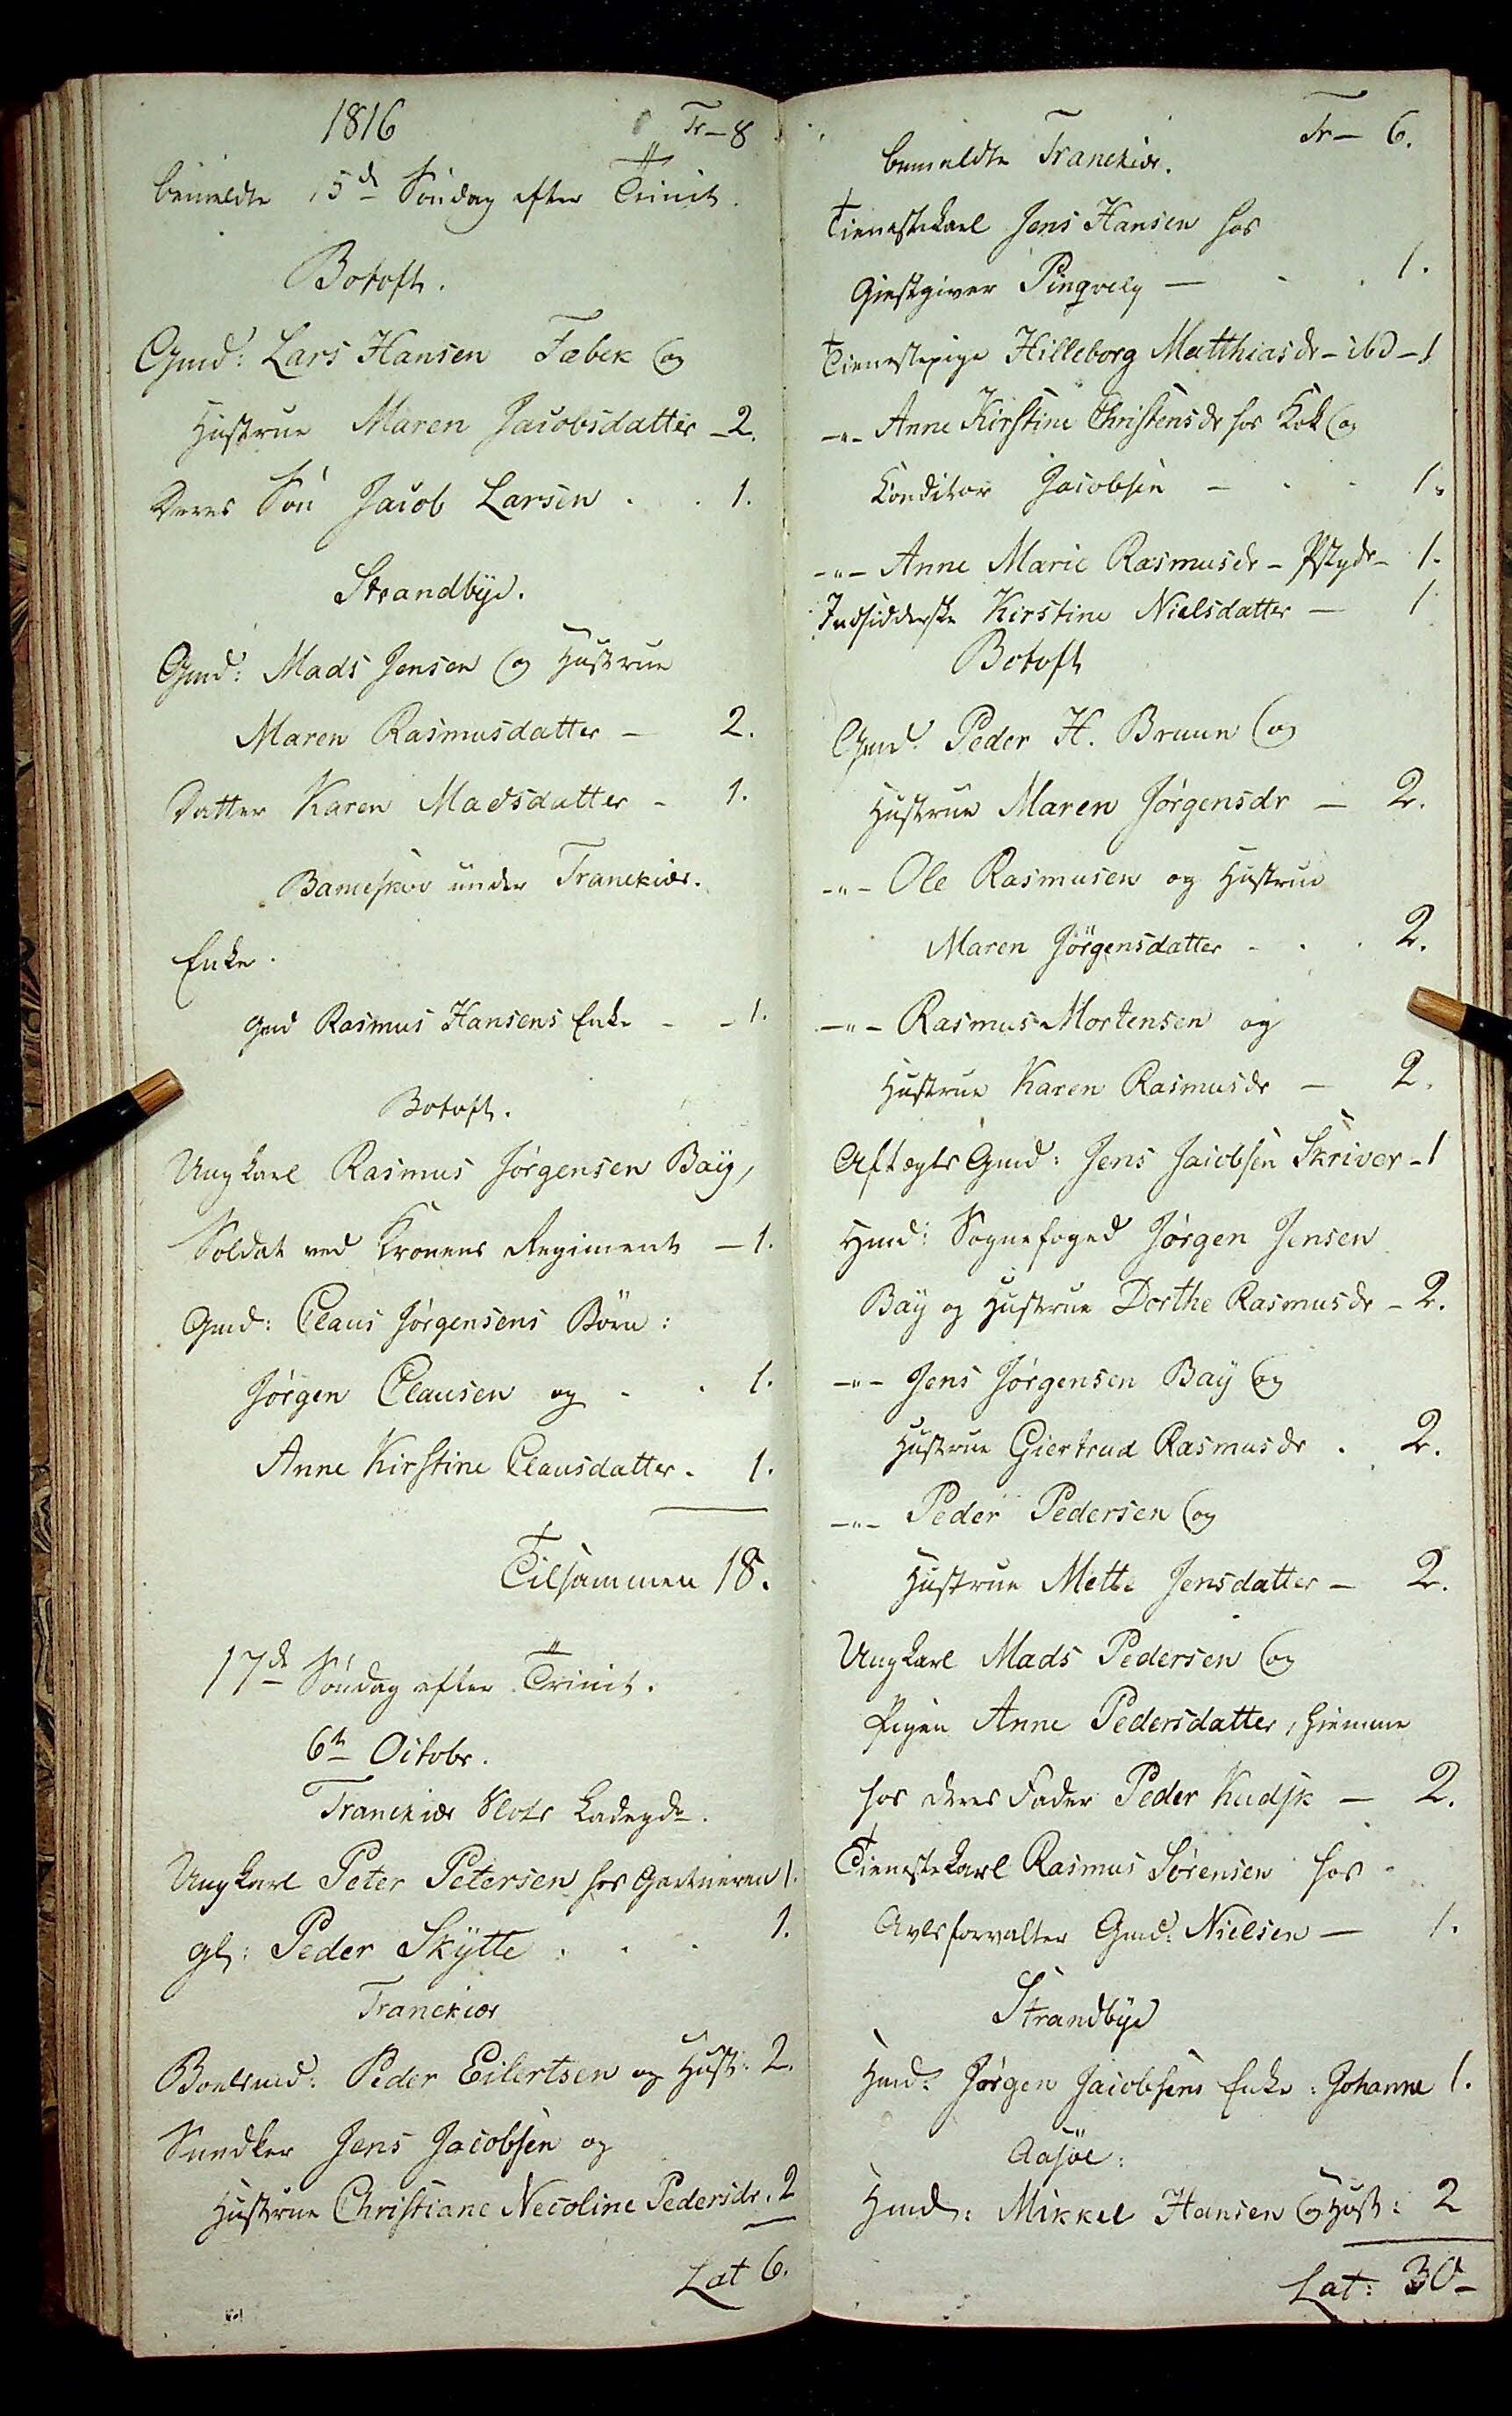1816
Tr - 8
bemeldte 15de Søndag efter Trinit.
Botoft.
Gmd: Lars Hansen Fæbek og
Hustrue Maren Jacobsdatter - 2.
Deres Søn Jacob Larsen . . 1.
Strandbye.
Gmd: Mads Jensen og Hustrue
Maren Rasmusdatter - 2.
Datter Karen Madsdatter - 1.
Bameskov under Tranekiær.
Enke
Gmd Rasmus Hansens Enke - - 1.
Botoft.
Ungkarl Rasmus Jørgensen Bay,
Soldat ved Konens Regiment - 1.
Gmd: Claus Jørgensens Børn:
Jørgen Clausen og . . 1.
Anne Kirstine Clausdatter . 1.
Tilsammen 18.
17de Søndag efter Trinit.
6te Octobr.
Tranekiær Slots Ladegdr
Ungkarl Peter Petersen hos Gartneren 1.
gl: Peder Skytte . . . 1.
Tranekiær
Boelsmd: Peder Eilertsen og Hust . 2.
Snedker Jens Jacobsen og
Hustrue Christiane Nicoline Pedersdr . 2
Lat 6.
Tr - 6.
bemeldte Tranekiær.
Tienestekarl Jens Hansen hos
Giestegiver Pingvely - . . 1.
Tienestepige Hilleborg Matthiasdr - ibd - 1
-"- Anne Kirstine Christensdr hos Kok og
Konditor Jacobsen - . . 1.
-"- Anne Marie Rasmusdr - Pstgdr 1.
Indsidderske Kirstine Nielsdatter - 1
Botoft
Gmd.  Peder H. Bruun og
Hustrue Maren Jørgensdr - 2.
-"- Ole Rasmusen og Hustrue
Maren Jørgensdatter . . . 2.
-"- Rasmus Mortensen og
Hustrue Karen Rasmusdr - 2.
Aftægts Gmd: Jens Jacobsen Skriver - 1
Hmd: Sognefoged Jørgen Jensen
Bay og Hustrue Dorthe Rasmusdr - 2.
-"- Jens Jørgensen Bay og
Hustrue Giertrud Rasmusdr . 2.
-"- Peder Pedersen og
Hustrue Mette Jensdatter - 2.
Ungkarl Mads Pedersen og
Pigen Anne Pedersdatter, hjemme
hos deres Fader Peder Kudsk - 2.
Tienestekarl Rasmus Sørensen hos
Avlsforvalter Gmd: Nielsen - 1.
Strandbye
Hmd: Jørgen Jacobsens Enke: Johanne 1.
Aasøe:
Hmd: Mikkel Hansen og Hust : 2
Lat: 30
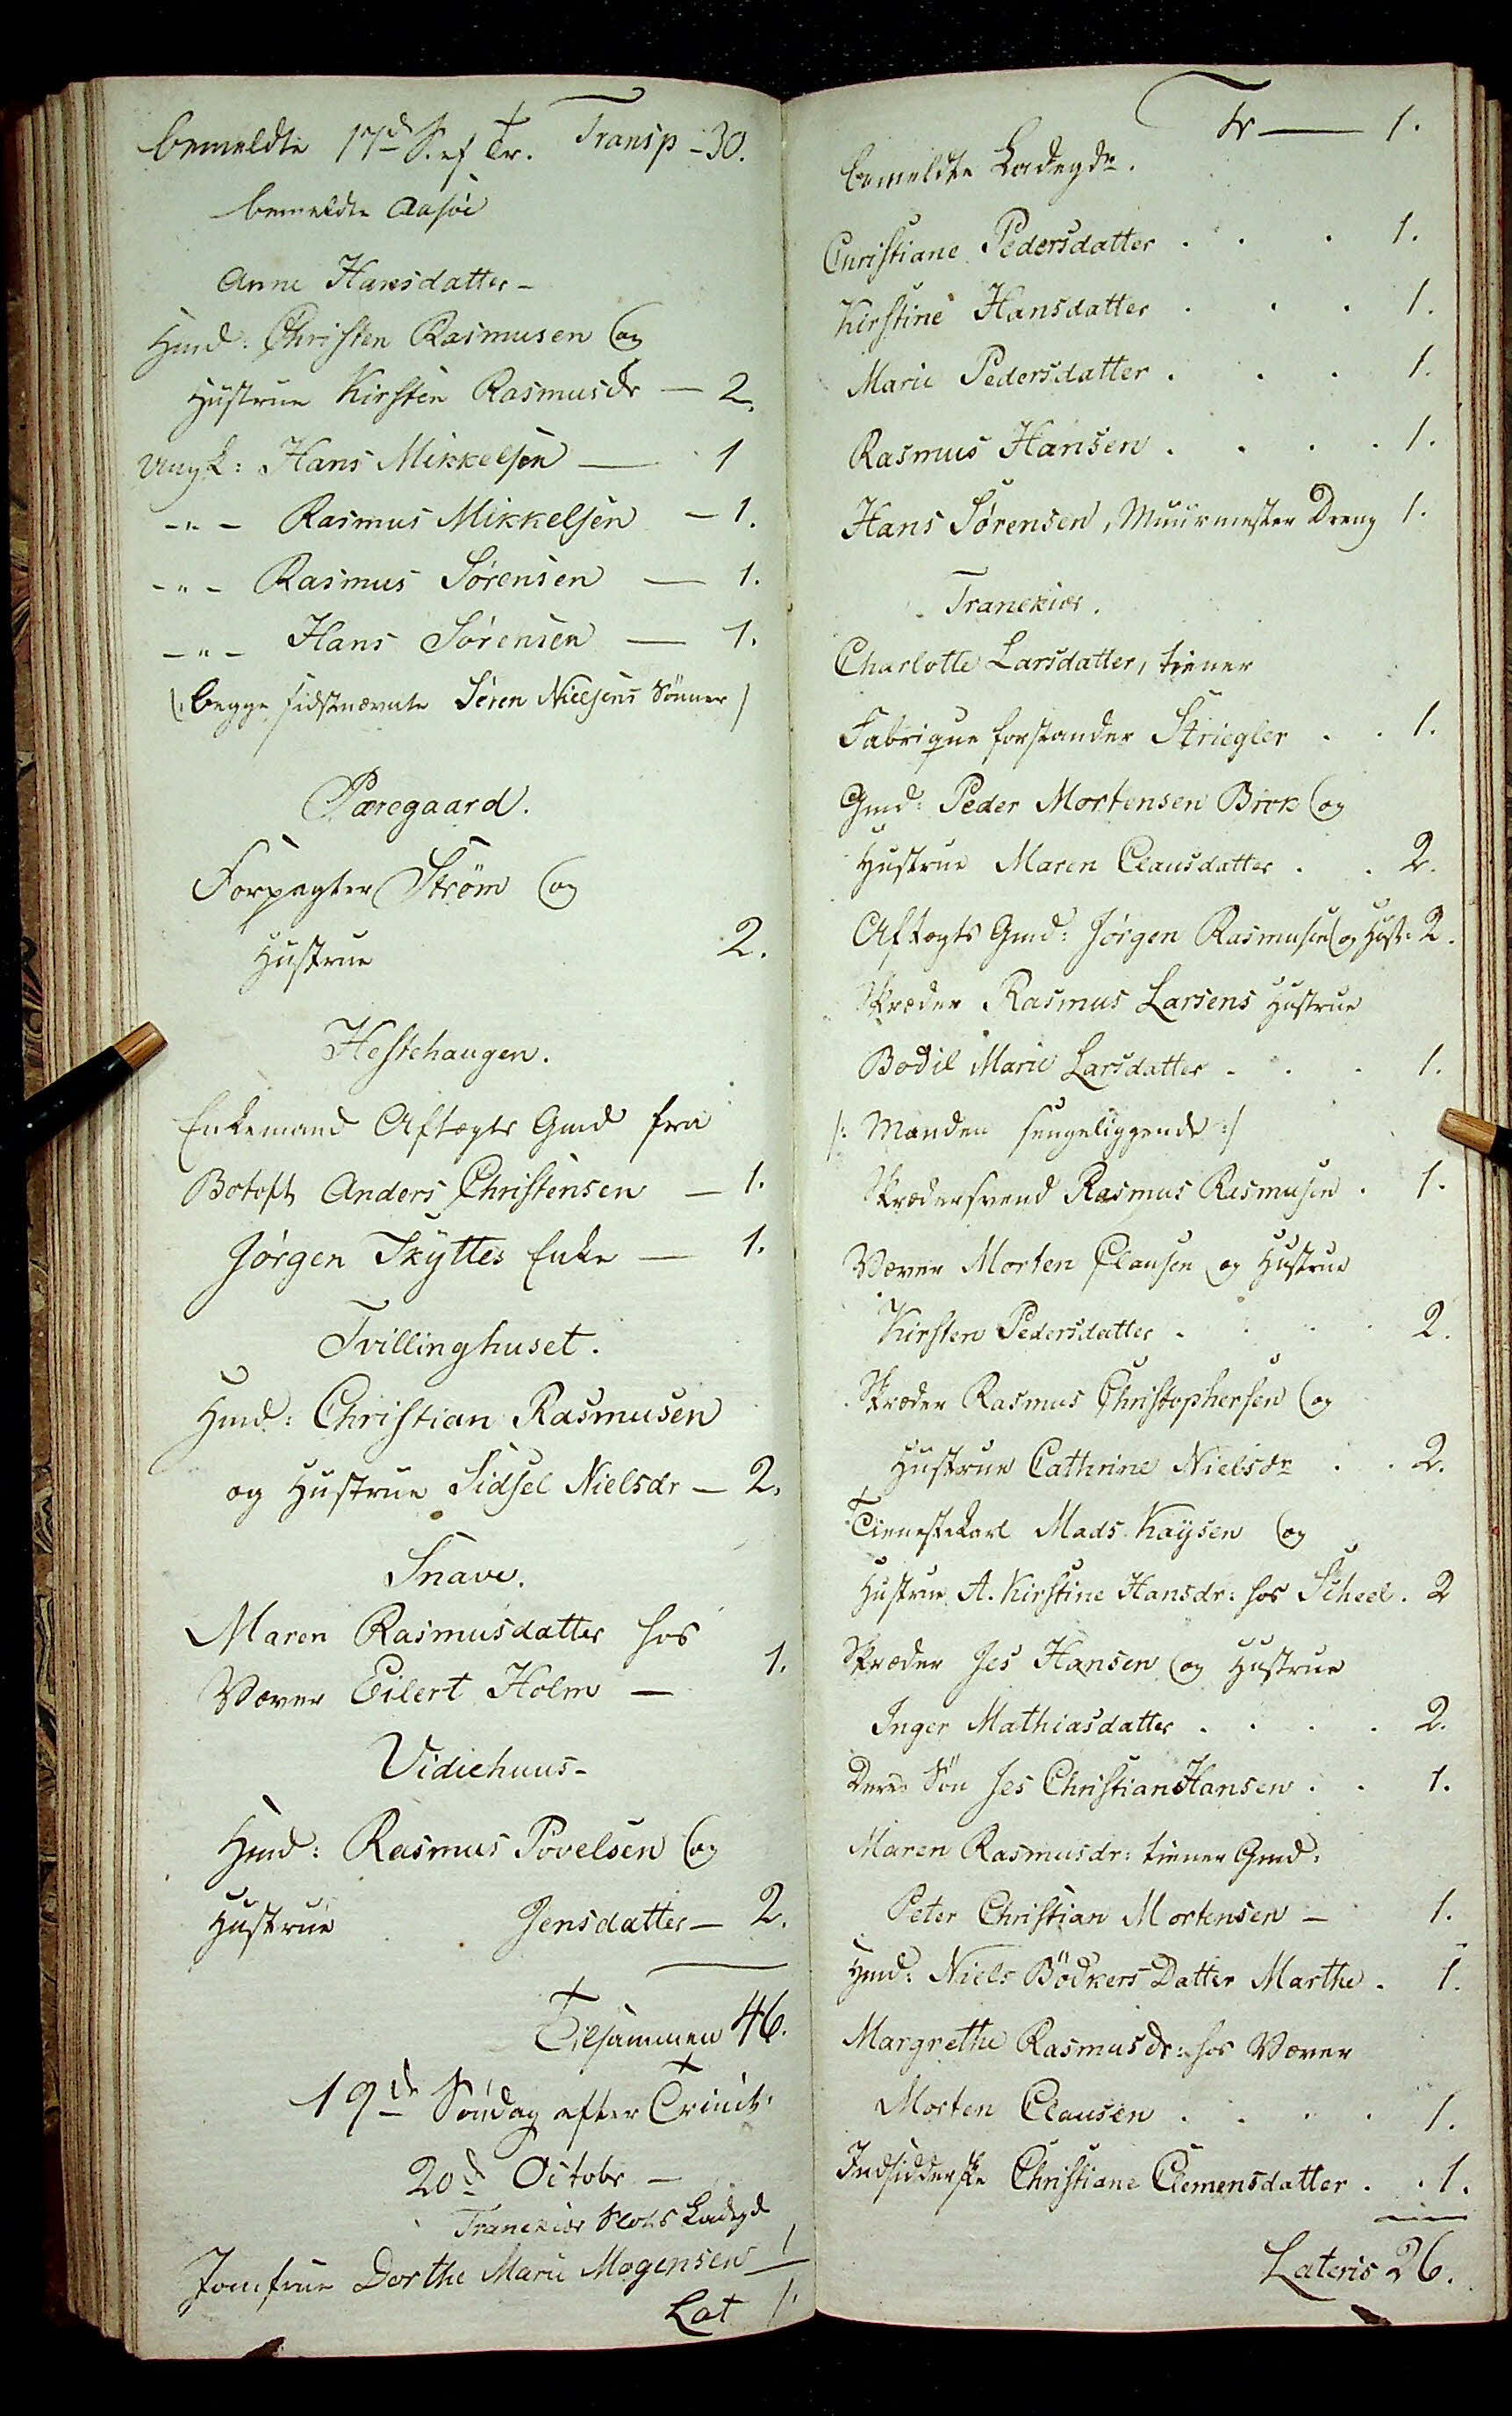Transp - 30.
bemeldte 17de S. ef Tr.
bemeldte Aasøe
Anne Hansdatter -
Hmd: Christen Rasmusen og
Hustrue Kirsten Rasmusdr - 2.
Ungk: Hans Mikkelsen - 1
-"- Rasmus Mikkelsen - 1.
-"- Rasmus Sørensen - 1.
-"- Hans Sørensen - 1.
|. begge sidstnævnte Søren Nielsens Sønner |
Pæregaard.
Forpagter Strøm og
Hustrue 2.
Hestehaugen.
Enkemand Aftægts Gmd fra
Botoft Anders Christensen -1.
Jørgen Skyttes Enke - 1.
Tvillinghuset.
Hmd: Christian Rasmusen
og Hustrue Sidsel Nielsdr - 2
Snave.
Maren Rasmusdatter hos
Væver Eilert Holm - 1.
Vidiehuus-
Hmd: Rasmus Povelsen og
Hustrue Jensdatter - 2.
Tilsammen 46
19de Søndag efter Trinit.
20de Octobr
Tranekiær Slots Ladedg
Jomfrue Dorthe Marie Mogensen 1
Lat 1
Tr - 1.
bemeldte Ladegdr.
Christiane Pedersdatter . . . 1.
Kirstine Hansdatter . . . 1.
Marie Pedersdatter . . . 1.
Rasmus Hansen . . . . 1.
Hans Sørensen, Muurmester Dreng 1.
Tranekiær.
Charlotte Larsdatter, tiener
Fabriqueforstander Striegler . . 1.
Gmd: Peder Mortensen Brok og
Hustrue Maren Clausdatter . . 2.
Aftægts Gmd: Jørgen Rasmusen og Hust . 2.
Skræder Rasmus Larsens Hustrue.
Bodil Marie Larsdatter . . . 1.
/: Manden sengeliggende :/
Skrædersvend Rasmus Rasmusen . 1.
Væver Morten Clausen og Hustrue
Kirsten Pedersdatter . . . . 2.
Skræder Rasmus Christophersen og
Hustrue Cathrine Nielsdr . . 2.
Tienestekarl Mads Kaysen og
Hustrue A. Kirstine Hansdr: hos Scheel . 2
Skræder Jes Hansen og Hustrue
Inger Mathiasdatter . . . . 2.
Deres Søn Jes Christian Hansen . . 1.
Maren Rasmusdr: tiener Gmd:
Peter Christian Mortensen - 1.
Hmd: Niels Bødkers Datter Marthe . 1.
Margrethe Rasmusdr: hos Væver
Morten Clausen . . . . 1.
Indsidderse Christiane Clemensdatter . . 1.
Lateris 26.